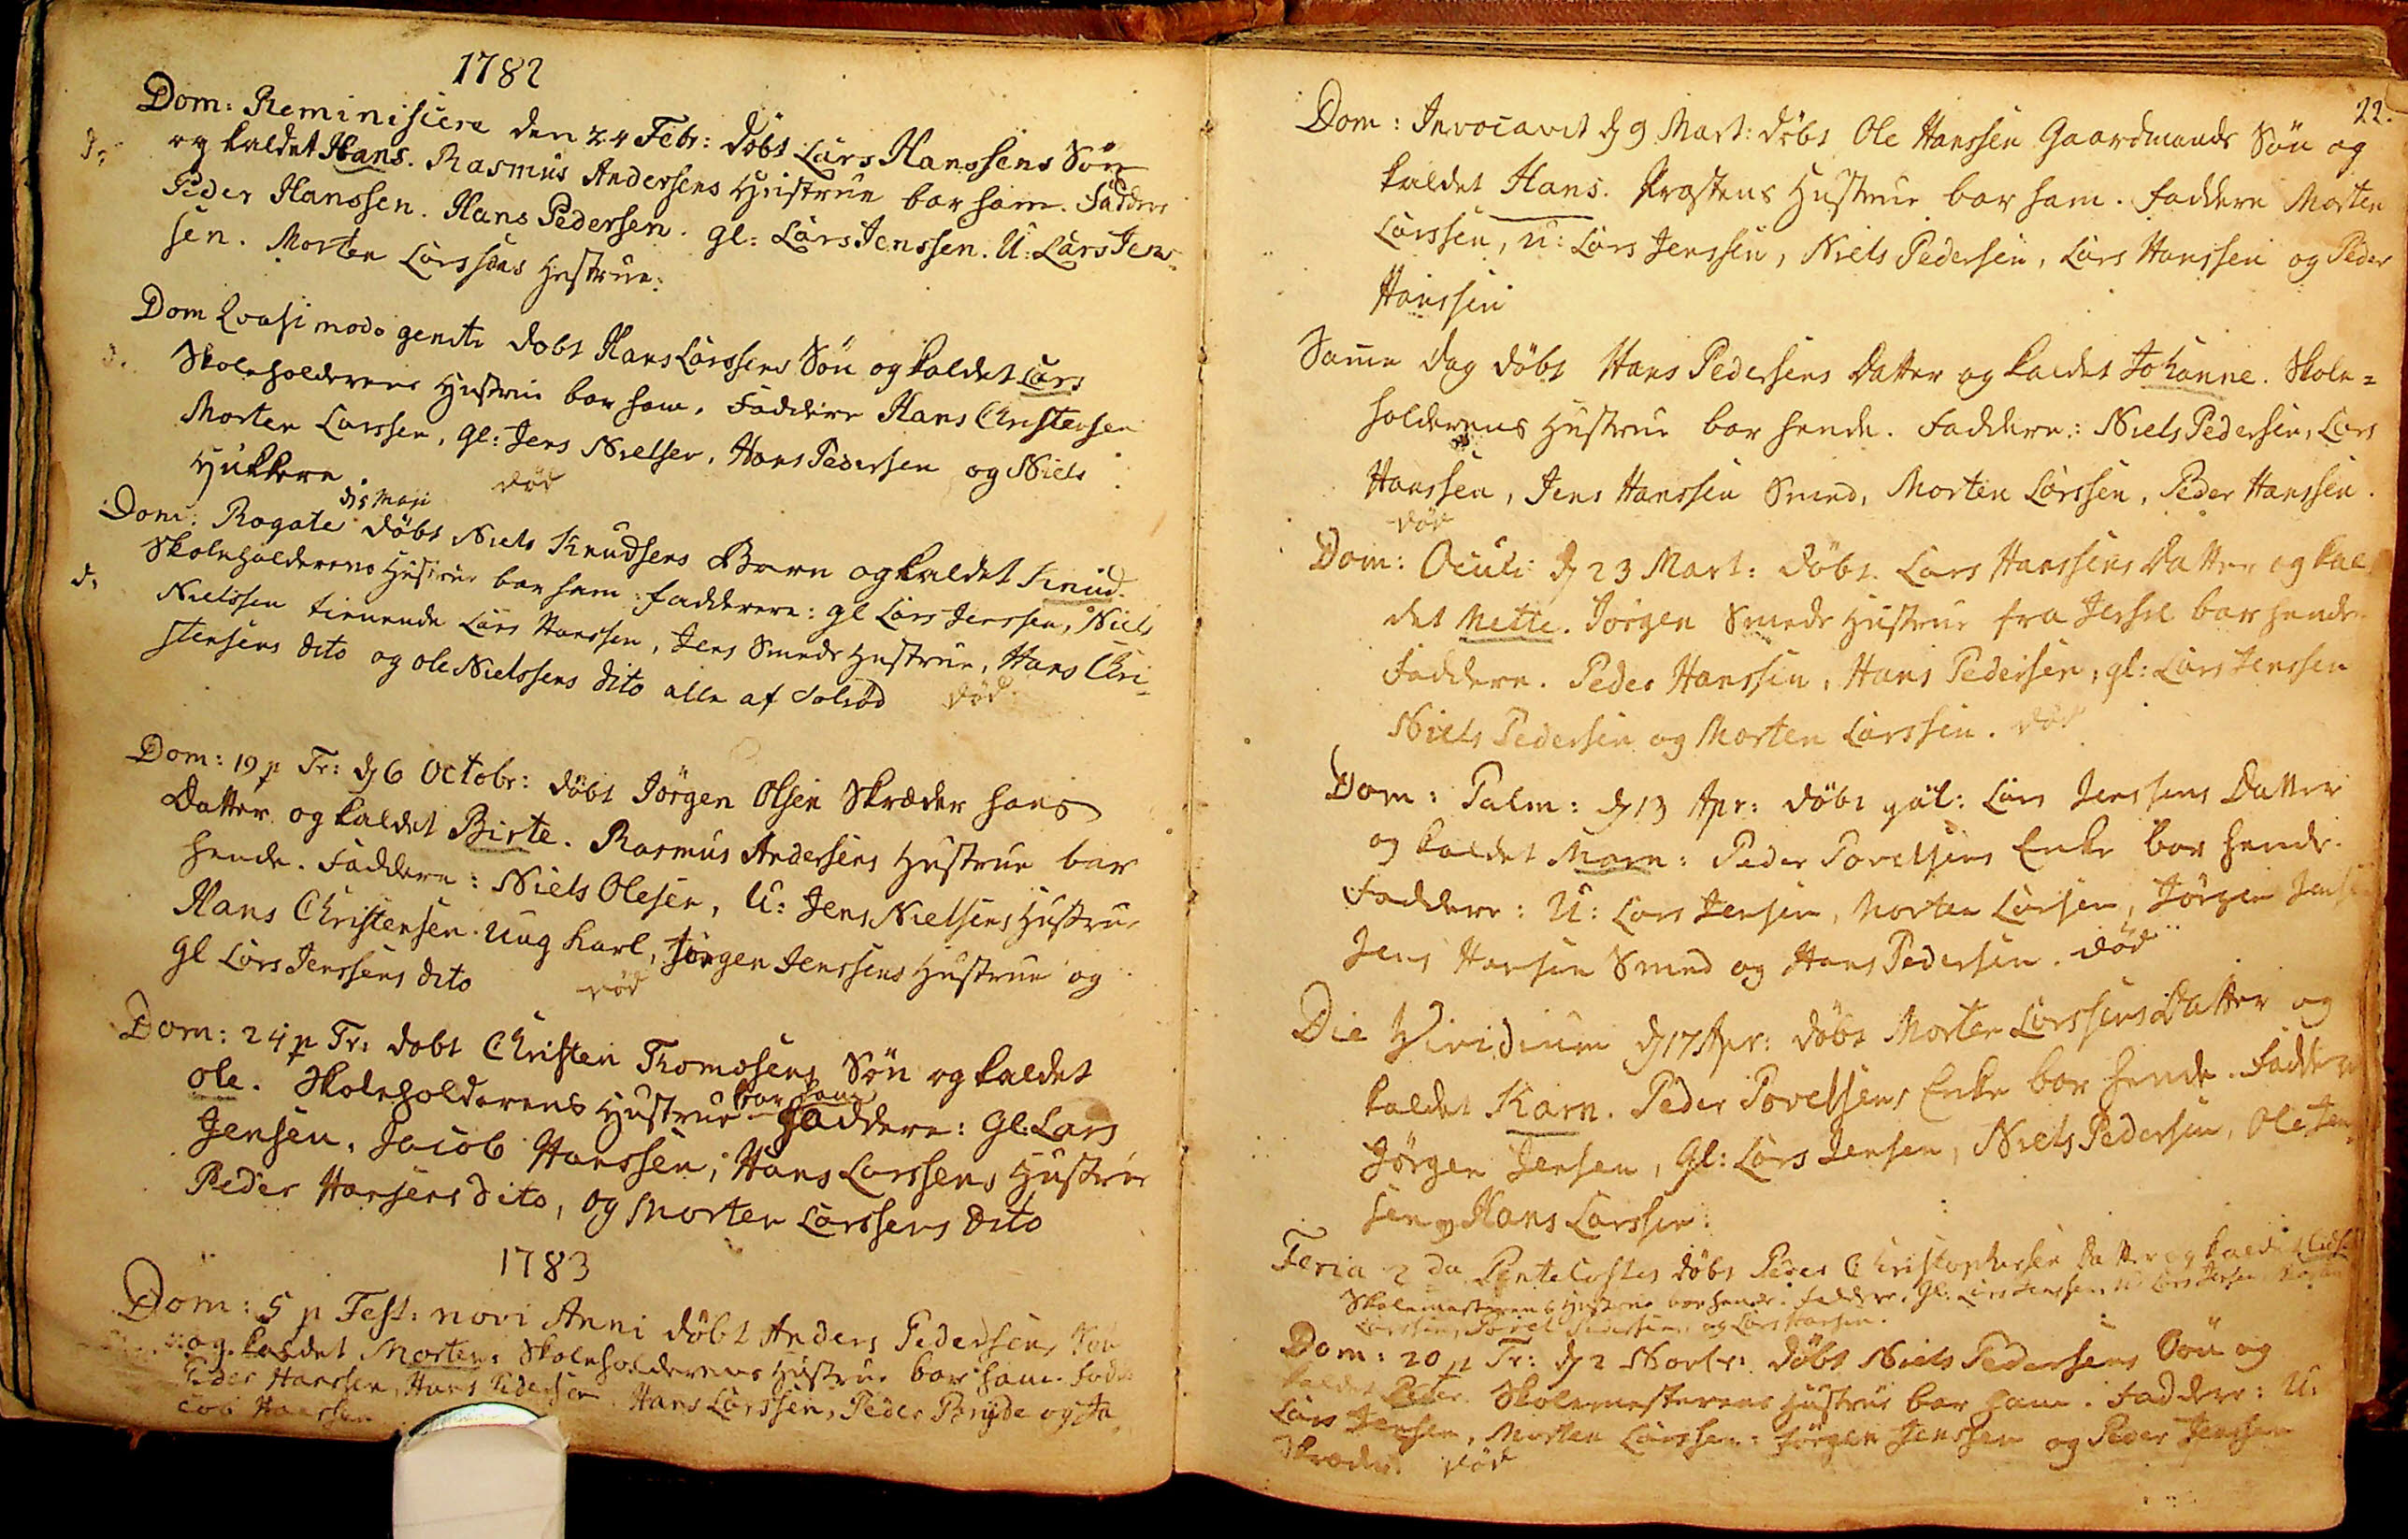1782
Dom: Reminiscere den 24 Febr: døbt Lars Hanssens Søn
og kaldet Hans. Rasmus Andersens Hustrue bar ham. Faddere
Peder Hanssen. Hans Pedersen. gl: Lars Jenssen. U: Lars Jens¬
sen. Morten Larssens Hustrue.
Dom Qvasimodo geniti døbt Hans Larssens Søn og kaldet Lars.
Skoleholderens Hustrue bar ham. Faddere Hans Christensen
Morten Larssen, gl: Jens Nielsen, Hans Pedersen og Niels
Hukker.
død
d 5 Maji
Dom: Rogate døbt Niels Knudsens Barn og kaldet Knud.
Skoleholderens Hustrue bar ham: Faddere:gl Lars Jenssen, Niels
Nielssen tienende Lars Hanssen, Jens Smeds Hustrue, Hans Chri¬
stensens dito og Ole Nielssens dito alle af Solrød død
Dom: 19 p Tr: d 6 Octobr: døbt Jørgen Olsen Skræder hans
Datter og kaldet Birte. Rasmus Andersens Hustrue bar
hende. Faddere: Niels Olesen, U: Jens Nielsens Hustrue,
Hans Christensen. Ung karl, Jørgen Jenssens Hustrue og
gl Lars Jenssens dito
død
Dom: 24 p Tr: døbt Christen Thomæsens Søn og kaldet
bar ham
Ole. Skoleholderens Hustrue Faddere: Gl: Lars
Jensen, Jacob Hanssen; Hans Larssens Hustrue
Peder Hansens dito, og Morten Larssens dito
1783
Dom: 5 p Fest: novi Anni døbt Anders Pedersens Søn
og kaldet Morten. Skoleholderens Hustrue bar ham. Fadd:
Peder Hanssen, Hans Pedersen, Hans Larssen, Peder Bryde og Ja¬
cob Hanssen.
22.
Dom: Invocavit d 9 Mart: døbt Ole Hanssen Gaardmands Søn og
kaldet Hans. Præstens Hustrue bar ham. Faddere Morten
Larssen, u:Lars Jenssen, Niels Pedersen, Lars Hanssen og Peder
Hanssen
Same Dag døbt Hans Pedersens Datter og kaldet Johanne. Skole¬
holderens Hustrue bar hende. Faddere: Niels Pedersen, Lars
Hanssen, Jens Hanssen Smed, Morten Larssen, Peder Hanssen.
død
Dom: Oculi d 23 Mart: døbt Lars Hanssens Datter og kal
det Mette. Jørgen Smeds Hustrue fra Jersie bar hende.
Faddere. Peder Hanssen, Hans Pedersen, gl: Lars Jenssen
Niels Pedersen og Morten Larssen.
død
Dom: Palm: d 13 Apr: døbt gml: Lars Jenssens Datter
og kaldet Marn. Peder Povelsens Enke bar hende.
Faddere: U: Lars Jensen, Morten Larsen, Jørgen Jensen
Jens Hansen Smed og Hans Pedersen. død
Die Wiridium d 17 Apr: døbt Morten Larssens Datter og
kaldet Karn. Peder Povelsens Enke bar hende. Faddere
Jørgen Jensen, Gl: Lars Jensen, Niels Pedersen, Ole Jen
sen og Hans Larssen.
Feria 2da Pentecostes døbt Peder Christophersen datter og kaldet Cidsel.
Skolemesterens Hustrue bar hende. Faddere: Gl. Lars Jenssen: U: Lars Jensen, Morten
Larssen, Povel Pedersen, og Lars Hansen.
Dom: 20 p Tr: d 2 Novbr: døbt Niels Pedersens Søn og
kaldet Peder. Skolemesterens Hustrue bar ham. Faddere. U:
Lars Jensen, Morten Larssen: Jørgen Jenssen og Peder Jenssen
Skræder. død
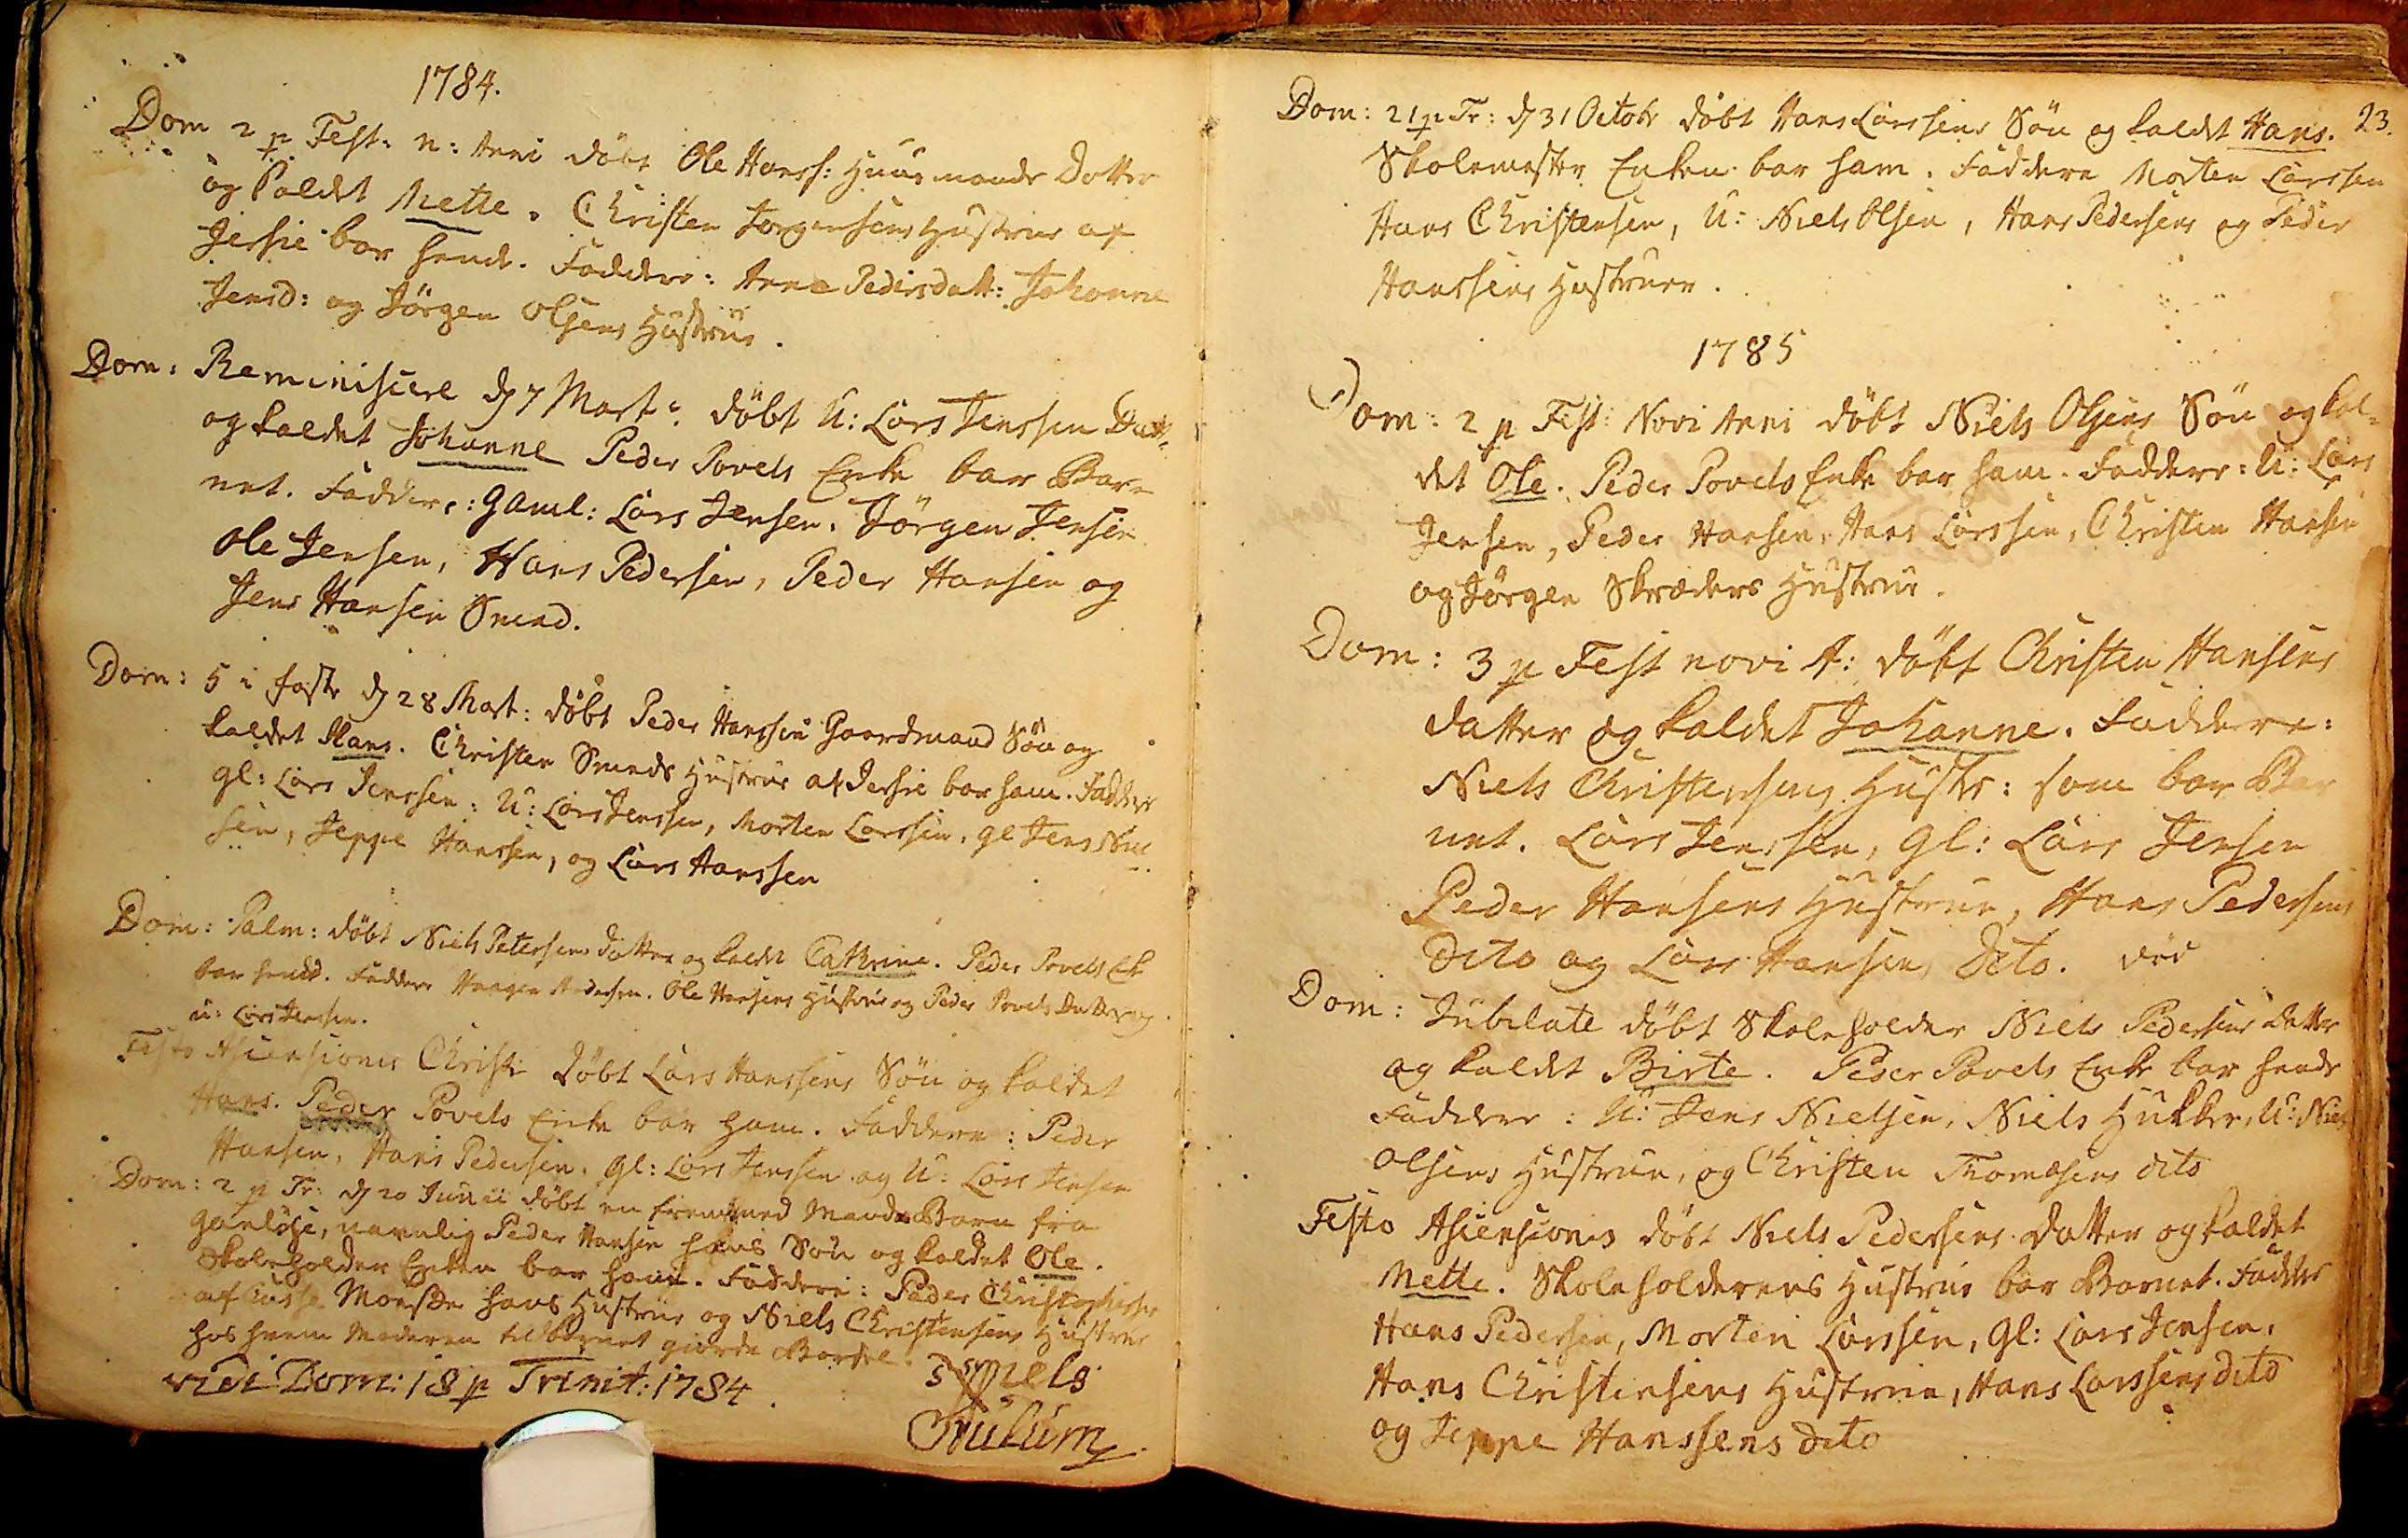1784.
Dom 2 p Fest: n: Anni døbt Ole Hanss: Huusmands Datter
og kaldet Mette. Christen Jørgensens Hustrue af
Jersie bar hende. Faddere: Anna Pedersdatt: Johanne
Jensd: og Jørgen Olsens Hustrue.
Dom: Reminiscere d 7 Mart: døbt U: Lars Jenssen Datter
og kaldet Johanne Peder Povels Enke bar Bar¬
net. Faddere: Gaml: Lars Jensen, Jørgen Jensen
Ole Jensen, Hans Pedersen, Peder Hansen og
Jens Hansen Smed.
Dom: 5 i Faste d 28 Mart: døbt Peder Hanssen Gaardmands Søn og
kaldet Hans. Christen Smeds Hustrue af Jersie bar ham. Faddere
gl:Lars Jenssen: U:Lars Jenssen, Morten Larssen, gl Jens Niel
sen, Jeppe Hanssen, og Lars Hanssen
Dom: Palm: døbt Niels Pedersens Datter og kaldet Cathrine. Peder Povels Enke
bar hende.Faddere Haagen Andersen,Ole Hanssens Hustrue og Peder Povels Datter og
u: Lars Jenssen.
Festo Ascensionis Christi døbt Lars Hanssens Søn og kaldet
Hans. Peder Povels Enke bar ham. Faddere: Peder
Hansen, Hans Pedersen, Gl: Lars Jenssen og U: Lars Jensen
Dom: 2 p Tr: d 20 Junii døbt en fremmed Mands Barn fra
Ganløse, nemlig Peder Hansen hans Søn og kaldet Ole.
Skoleholderens Enke bar ham. Faddere: Peder Christophersen
af Kasse## Moesse## hans Hustrue og Niels Christensens Hustrue
hos hvem Moderen til barnet giorde Barsel.
vidi Dom: 18 p Trinit: 1784.
Niels
Aulum
23.
Dom: 21 p Tr: d 31 Octobr døbt Hans Larssens Søn og kaldet Hans.
Skolemester Enken bar ham. Faddere Morten Larssen
Hans Christensen, U: Niels Olsen, Hans Pedersens og Peder
Hanssens Hustruer.
1785
Dom: 2 p Fest: Novi Anni døbt Niels Olsens Søn og kal¬
det Ole. Peder Povels Enke bar ham. Faddere: U: Lars
Jensen, Peder Hansen, Hans Larssen, Christen Hansen
og Jørgen Skræders Hustrue.
Dom: 3 p Fest novi A: døbt Christen Hansens
Datter og kaldet Johanne. Faddere:
Niels Christensens Hustr: som bar Bar
net. Lars Jenssen, gl: Lars Jensen
Peder Hansens Hustrue, Hans Pedersens
dito og Lars Hansens dito. død
Dom: Jubilate døbt Skoleholder Niels Pedersens Datter
og kaldet Birte. Peder Povels Enke bar hende
Faddere: U: Jens Nielsen, Niels Hukker, U: Niels
Olsens Hustrue, og Christen Thomæsens dito
Festo Ascensionis døbt Niels Pedersens Datter og kaldet
Mette. Skoleholderens Hustrue bar Barnet. Faddere
Hans Pedersen, Morten Larssen, Gl: Lars Jensen,
Hans Christensens Hustrue, Hans Larssens dito
og Jeppe Hanssens dito